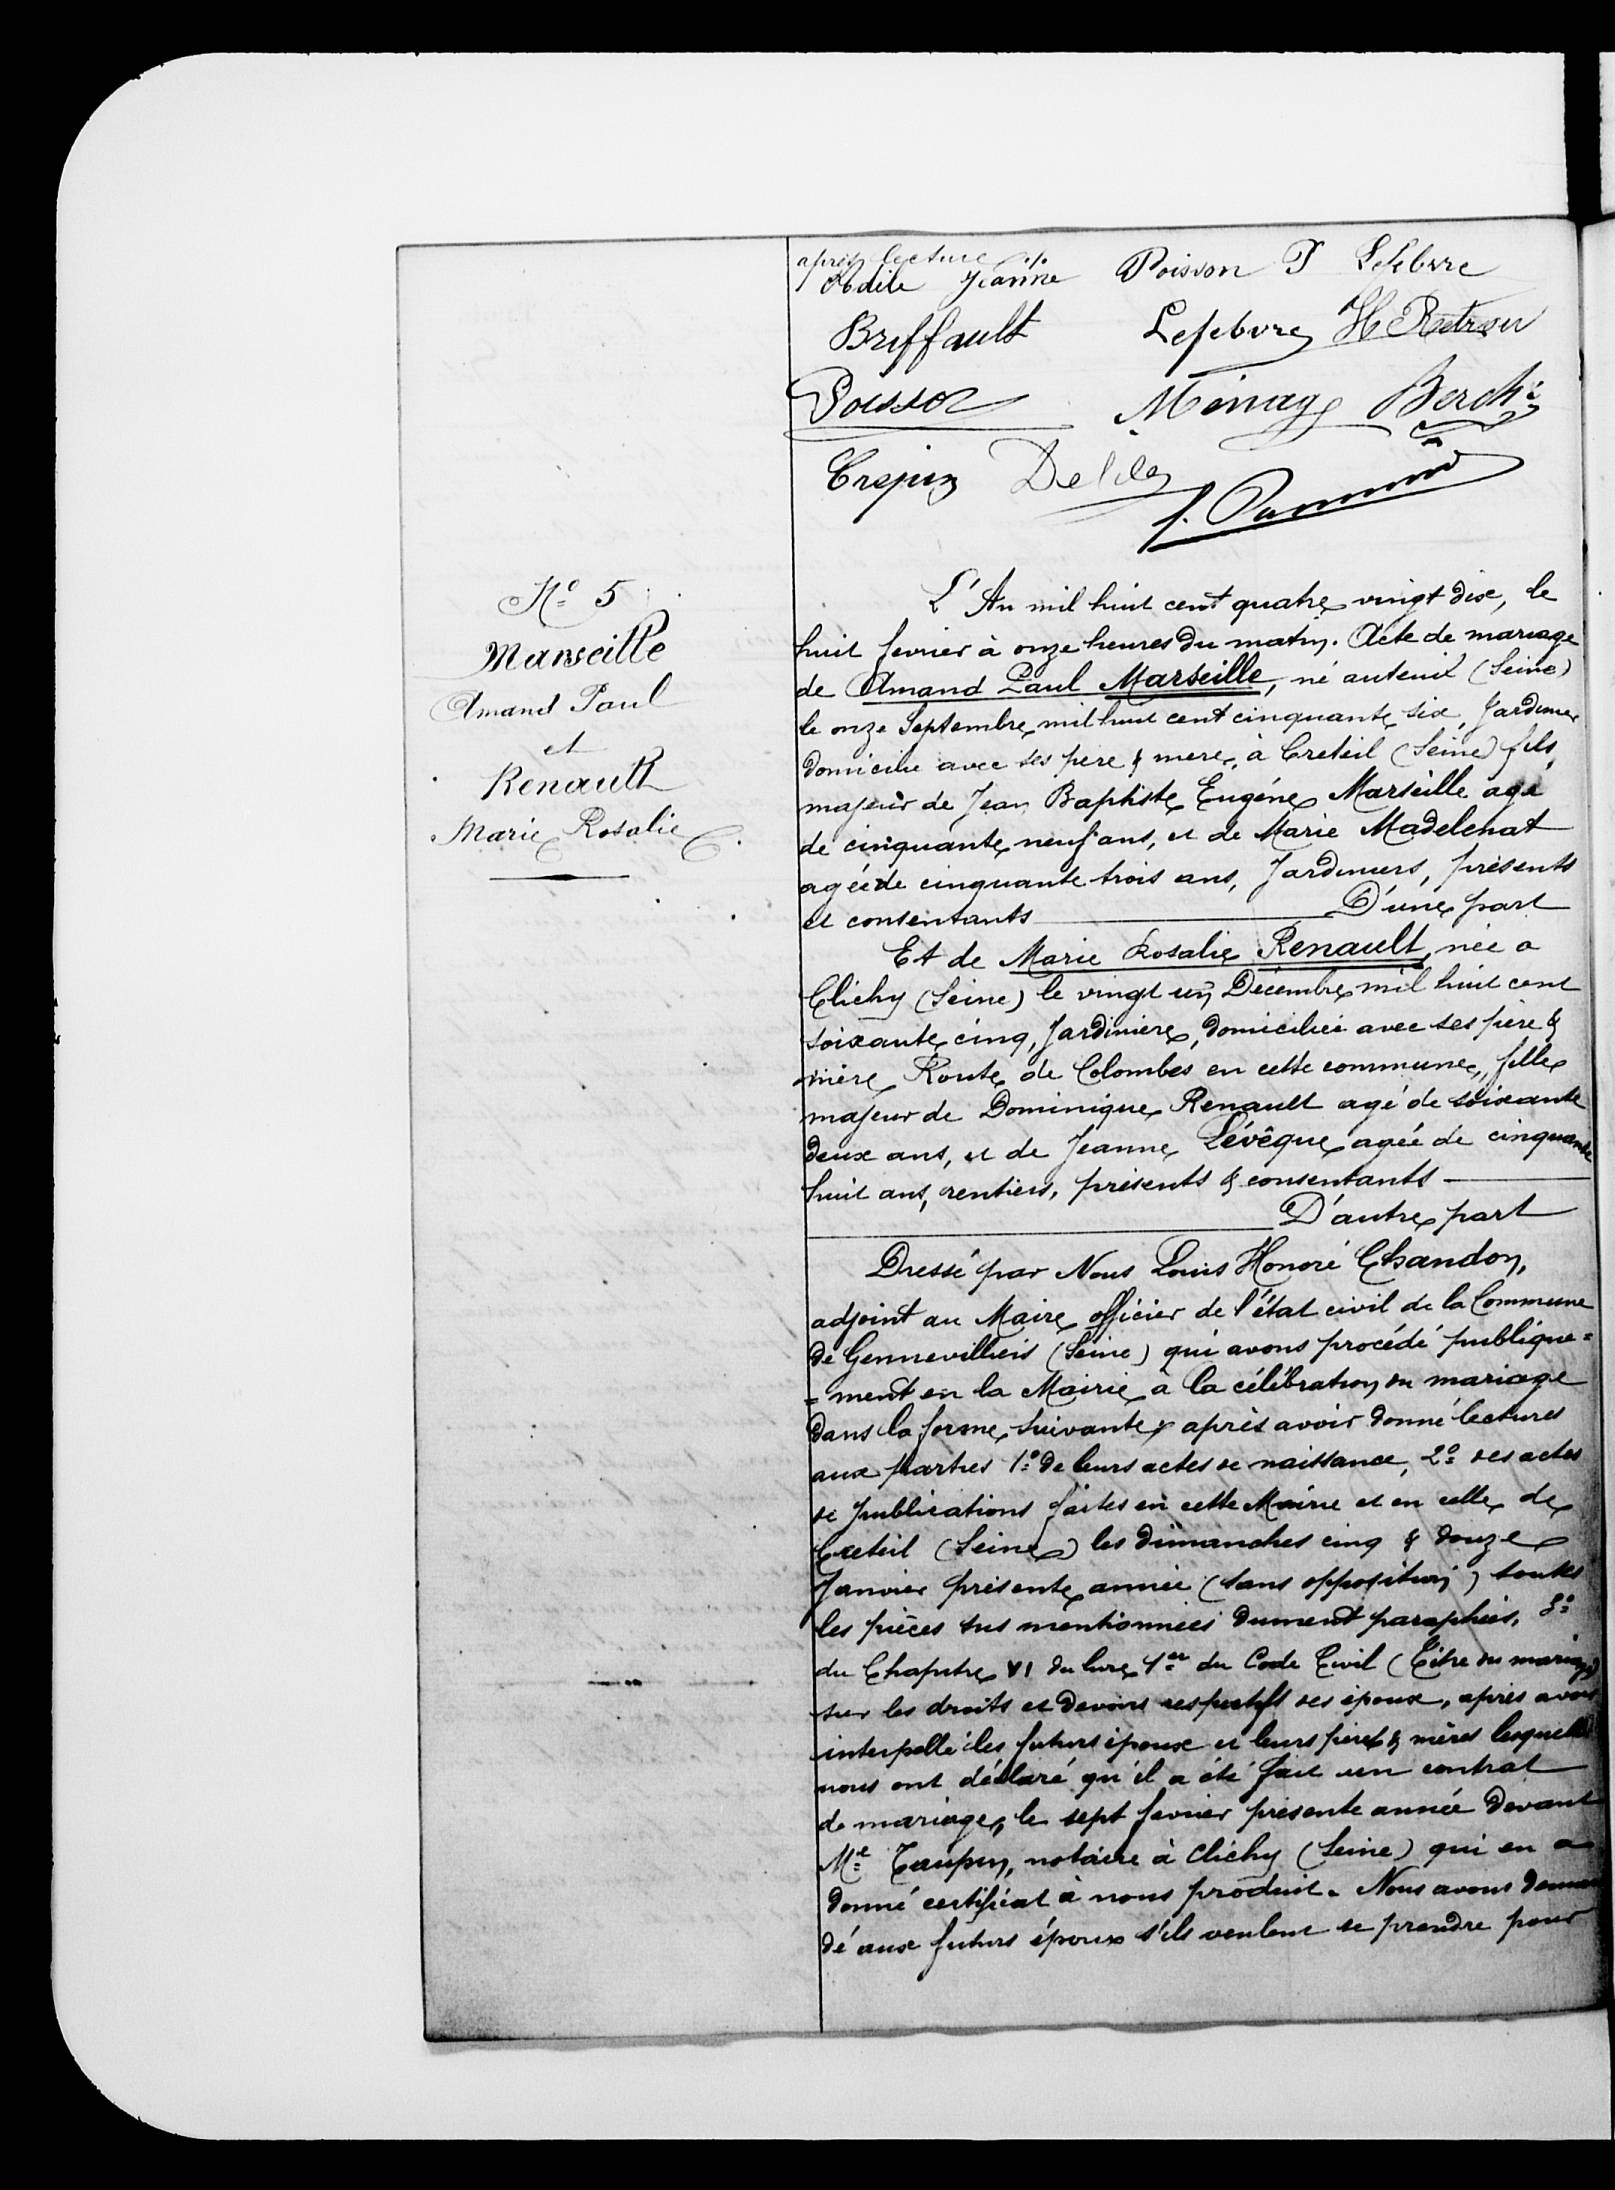N°5
Marseille
Armand Paul
et
Renault
Marie Rosalie
L'an mil huit cent quatre vingt dix, le
huit février à onze heures du matin. Acte de mariage
de Armand Paul Marseille, né auteuil (Seine)
le onze septembre mil huit cent cinquante six, jardinier
domicilié avec ses père & mère à Créteil (Seine) fils
majeur de Jean Baptiste Eugène Marseille âgé
de cinquante neuf ans, et de Marie Madelenat
agée de cinquante trois ans, Jardiniers, présents
et consentants -D'une part
Et de Marie Rosalie Renault, née à
Clichy (Seine) le vingt un Décembre mil huit cent
soixante cinq, jardiniere, domiciliée avec ses père &
mère, route de Colombes en cette commune, fille
majeur de Dominique Renault agé de soixante
deux ans, et de Jeanne Lévêque, agée de cinquante
huit ans, rentiers, présents & consentants -
-D'autre part
Dressé par Nous Louis Honoré Chandon,
adjoint au Maire officier de l'état civil de la Commune
de Gennevilliers (Seine) qui avons procédé publique-
-ment en la Mairie, à la célébration du mariage
dans la forme suivante après avoir donné lecture
aux parties 1°/ de leurs actes de naissance, 2°/ des actes
de publications faites en cette Mairie et en celle de
Créteil (Seine) les dimanches cinq & douze
janvier présente année (sans opposition) toutes
les pièces sus mentionnées dument paraphées, 3°/
du Chapitre VI du livre 1er du Code Civil (Titre du mariage)
sur les droits et devoirs respectifs des époux, après avoir
interpellé les futurs époux et leurs pères et mères lesquels
nous ont déclaré qu'il a été fait un contrat
de mariage, le sept février présente année devant
Me Taupen, notaire à Clichy (Seine) qui en a
donné certificat à nous produire. Nous avons deman
dé aux futurs époux s'ils veulent se prendre pour
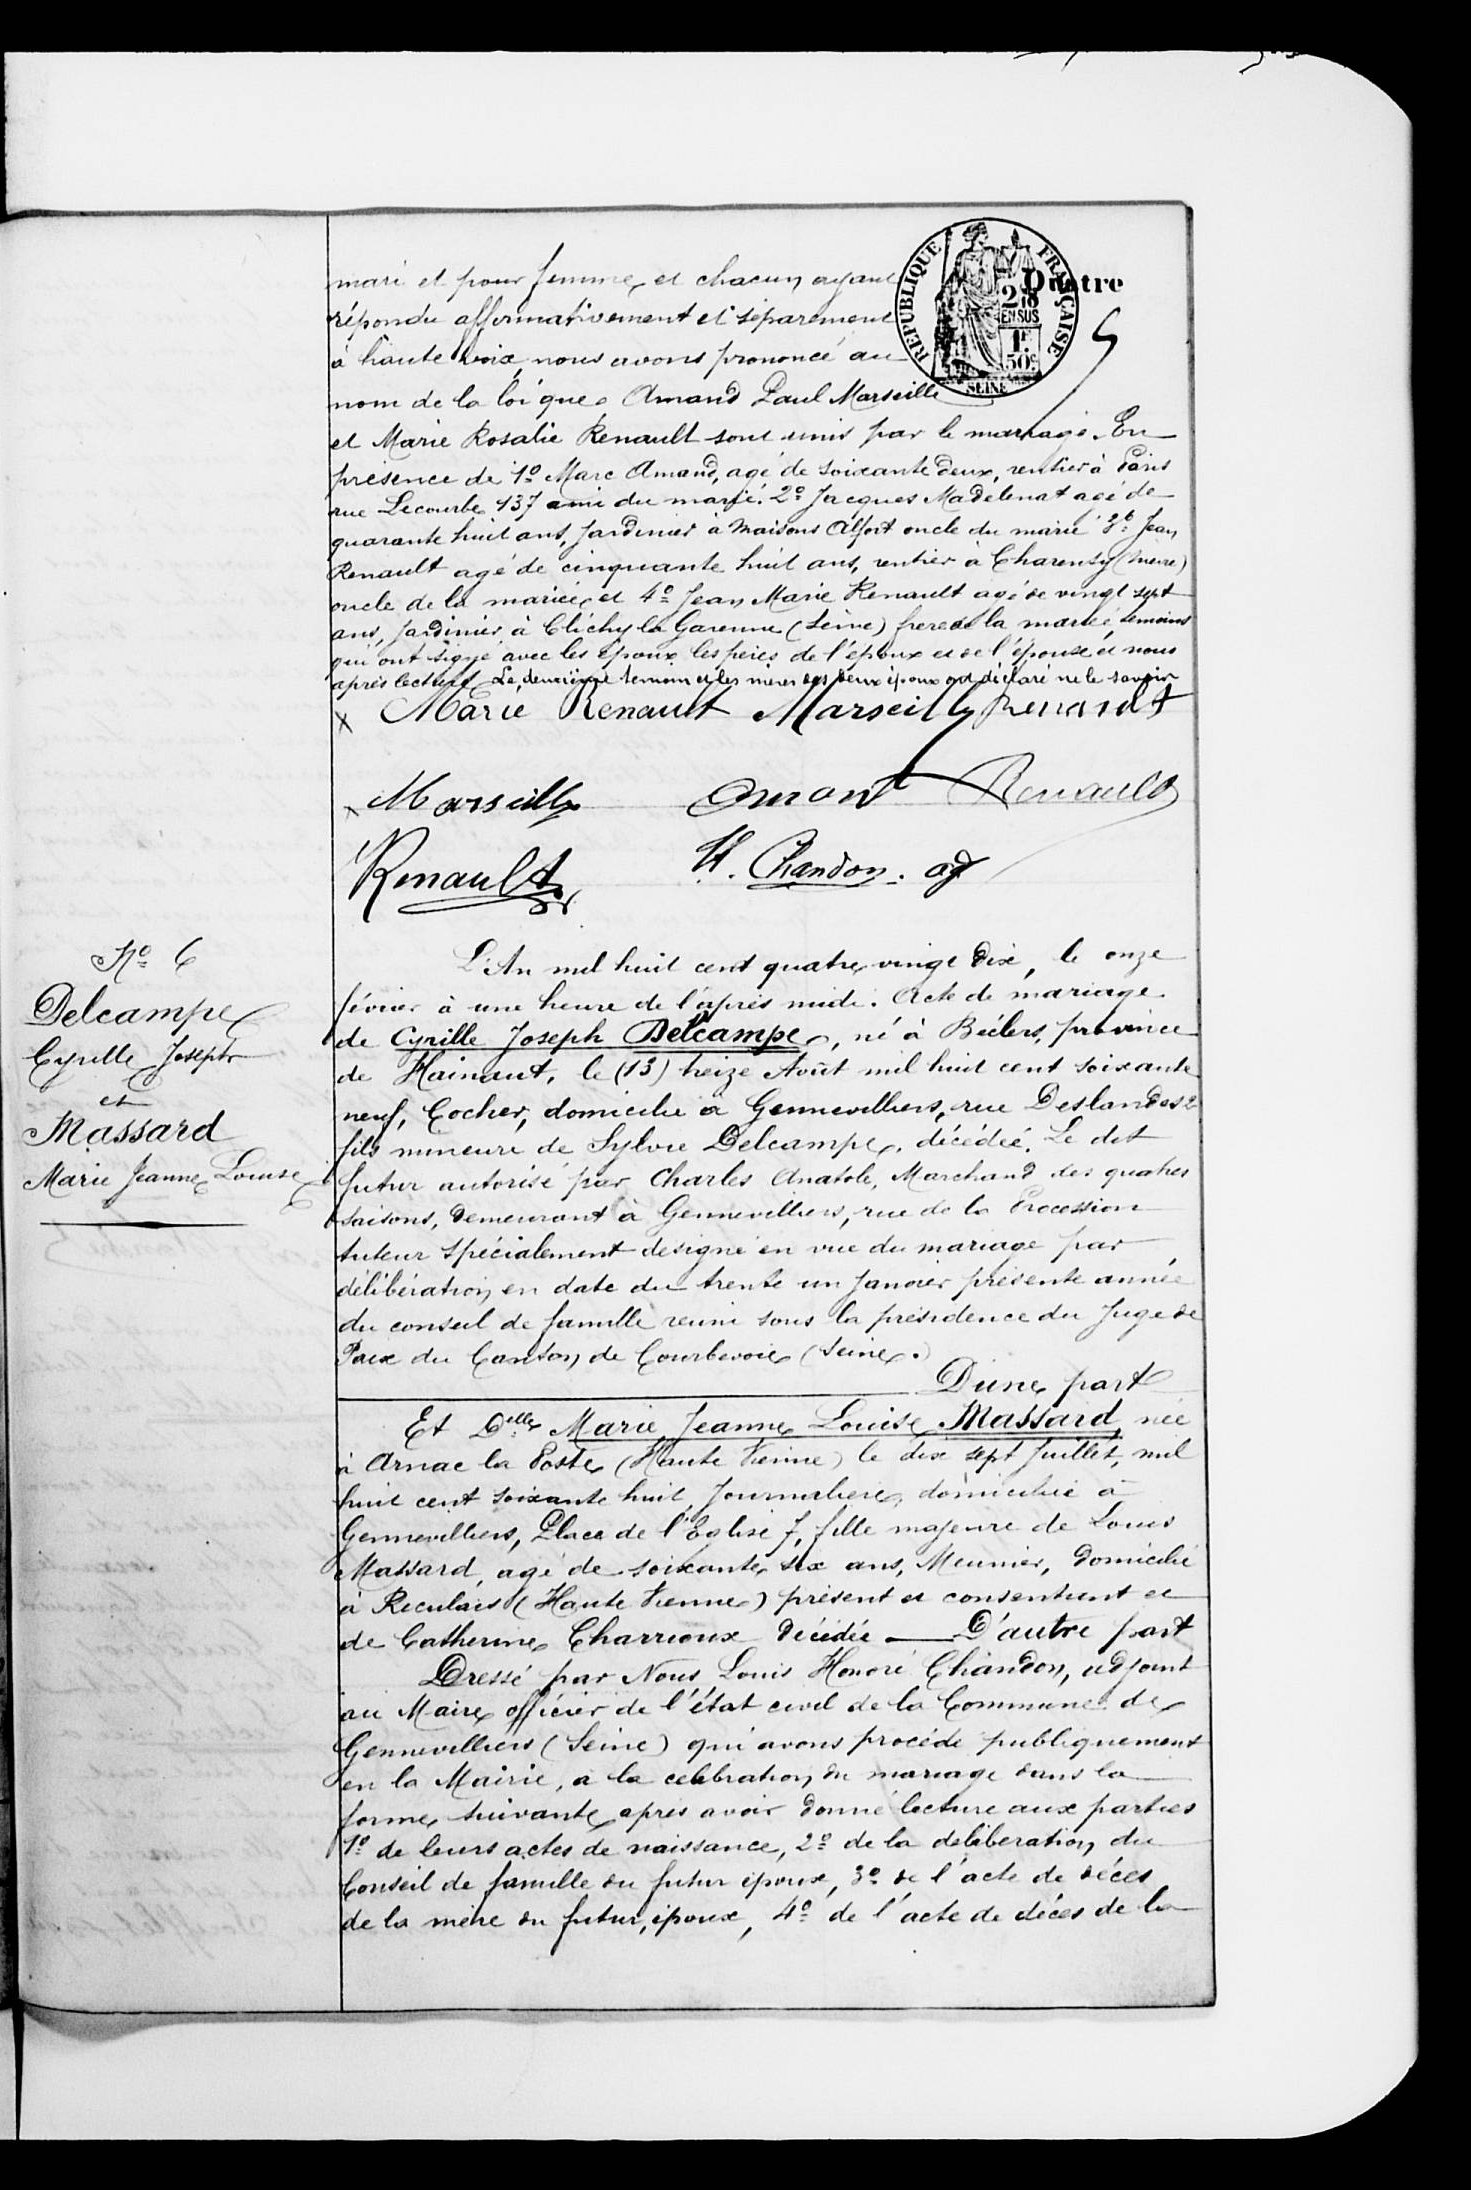mari et pour femme, et chacun ayant
répondu affirmativement et séparement
à haute voix nous avons prononcé au
nom de la loi que Arnaud Paul Marseille
et Marie Rosalie Renault sont unis par le mariage. En
présence de 1°: Marc Arnaud, agé de soixante deux, rentier à Paris
rue Lecourbe 137 ami du marié. 2°: Jacques Madelenat agé de
quarante huit ans, jardinier à Maisons Alfort oncle du marié 3°: Jean
Renault agé de cinquante huit ans, rentier à Charensy (seine)
oncle de la mariée et 4°: Jean Marie Renault agé de vingt sept
ans, jardinier à Clichy la Garenne (Seine) frère de la mariée, témoins
qui ont signé avec les époux les pères de l'époux et de l'épouse et nous
après lecture le deuxième témoin et les mère des deux époux ont déclaré ne le savoir
N°6
Delcampe
Cyrille Joseph
et
Massard
Marie Jeanne Louise
L'An mil huit cent quatre vingt dix, le onze
février à une heure de l'après midi. Acte de mariage
de Cyrille Joseph Delcampe, né à Béclers, province
de Hainaut, le (13) treize Août mil huit cent soixante
neuf, Cocher, domicilié à Gennevilliers, rue Deslandes
fils mineur de Sylvie Delcampe, décédé Le dit
futur autorisé par Charles Anatole, Marchand des quatres
saisons, demeurant à Gennevilliers, rue de la Procession
tuteur spécialement désigné en vue du mariage par
délibération en date du trente un janvier présente année
du conseil de famille réuni sous la présidence du Juge de
paix du Canton de Courbevoie (Seine.)
-D'une part
Et Delle Marie Jeanne Louise Massard, née
à Arnac la Poste (Haute Vienne) le dix sept juillet, mil
huit cent soixante huit, journalière domicilié à
Gennevilliers, Place de l'Eglise 7, fille majeure de Louis
Massard, agé de soixante six ans, Meunier, domicilié
à Reculaix (Haute Vienne) présent et consentant et
de Catherine Charroux décédée -D'autre part
Dressé par Nous Louis Honoré Chandon, adjoint
au Maire officier de l'état civil de la Commune de
Gennevilliers (Seine) qui avons procédé publiquement
en la Mairie, à la célébration du mariage dans la
forme suivante après avoir donné lecture aux parties
1°: de leurs actes de naissance, 2°: de la délibération, du
Conseil de famille du futur époux, 3°: de l'acte de décès
de la mère du futur époux, 4°: de l'acte de décès de la